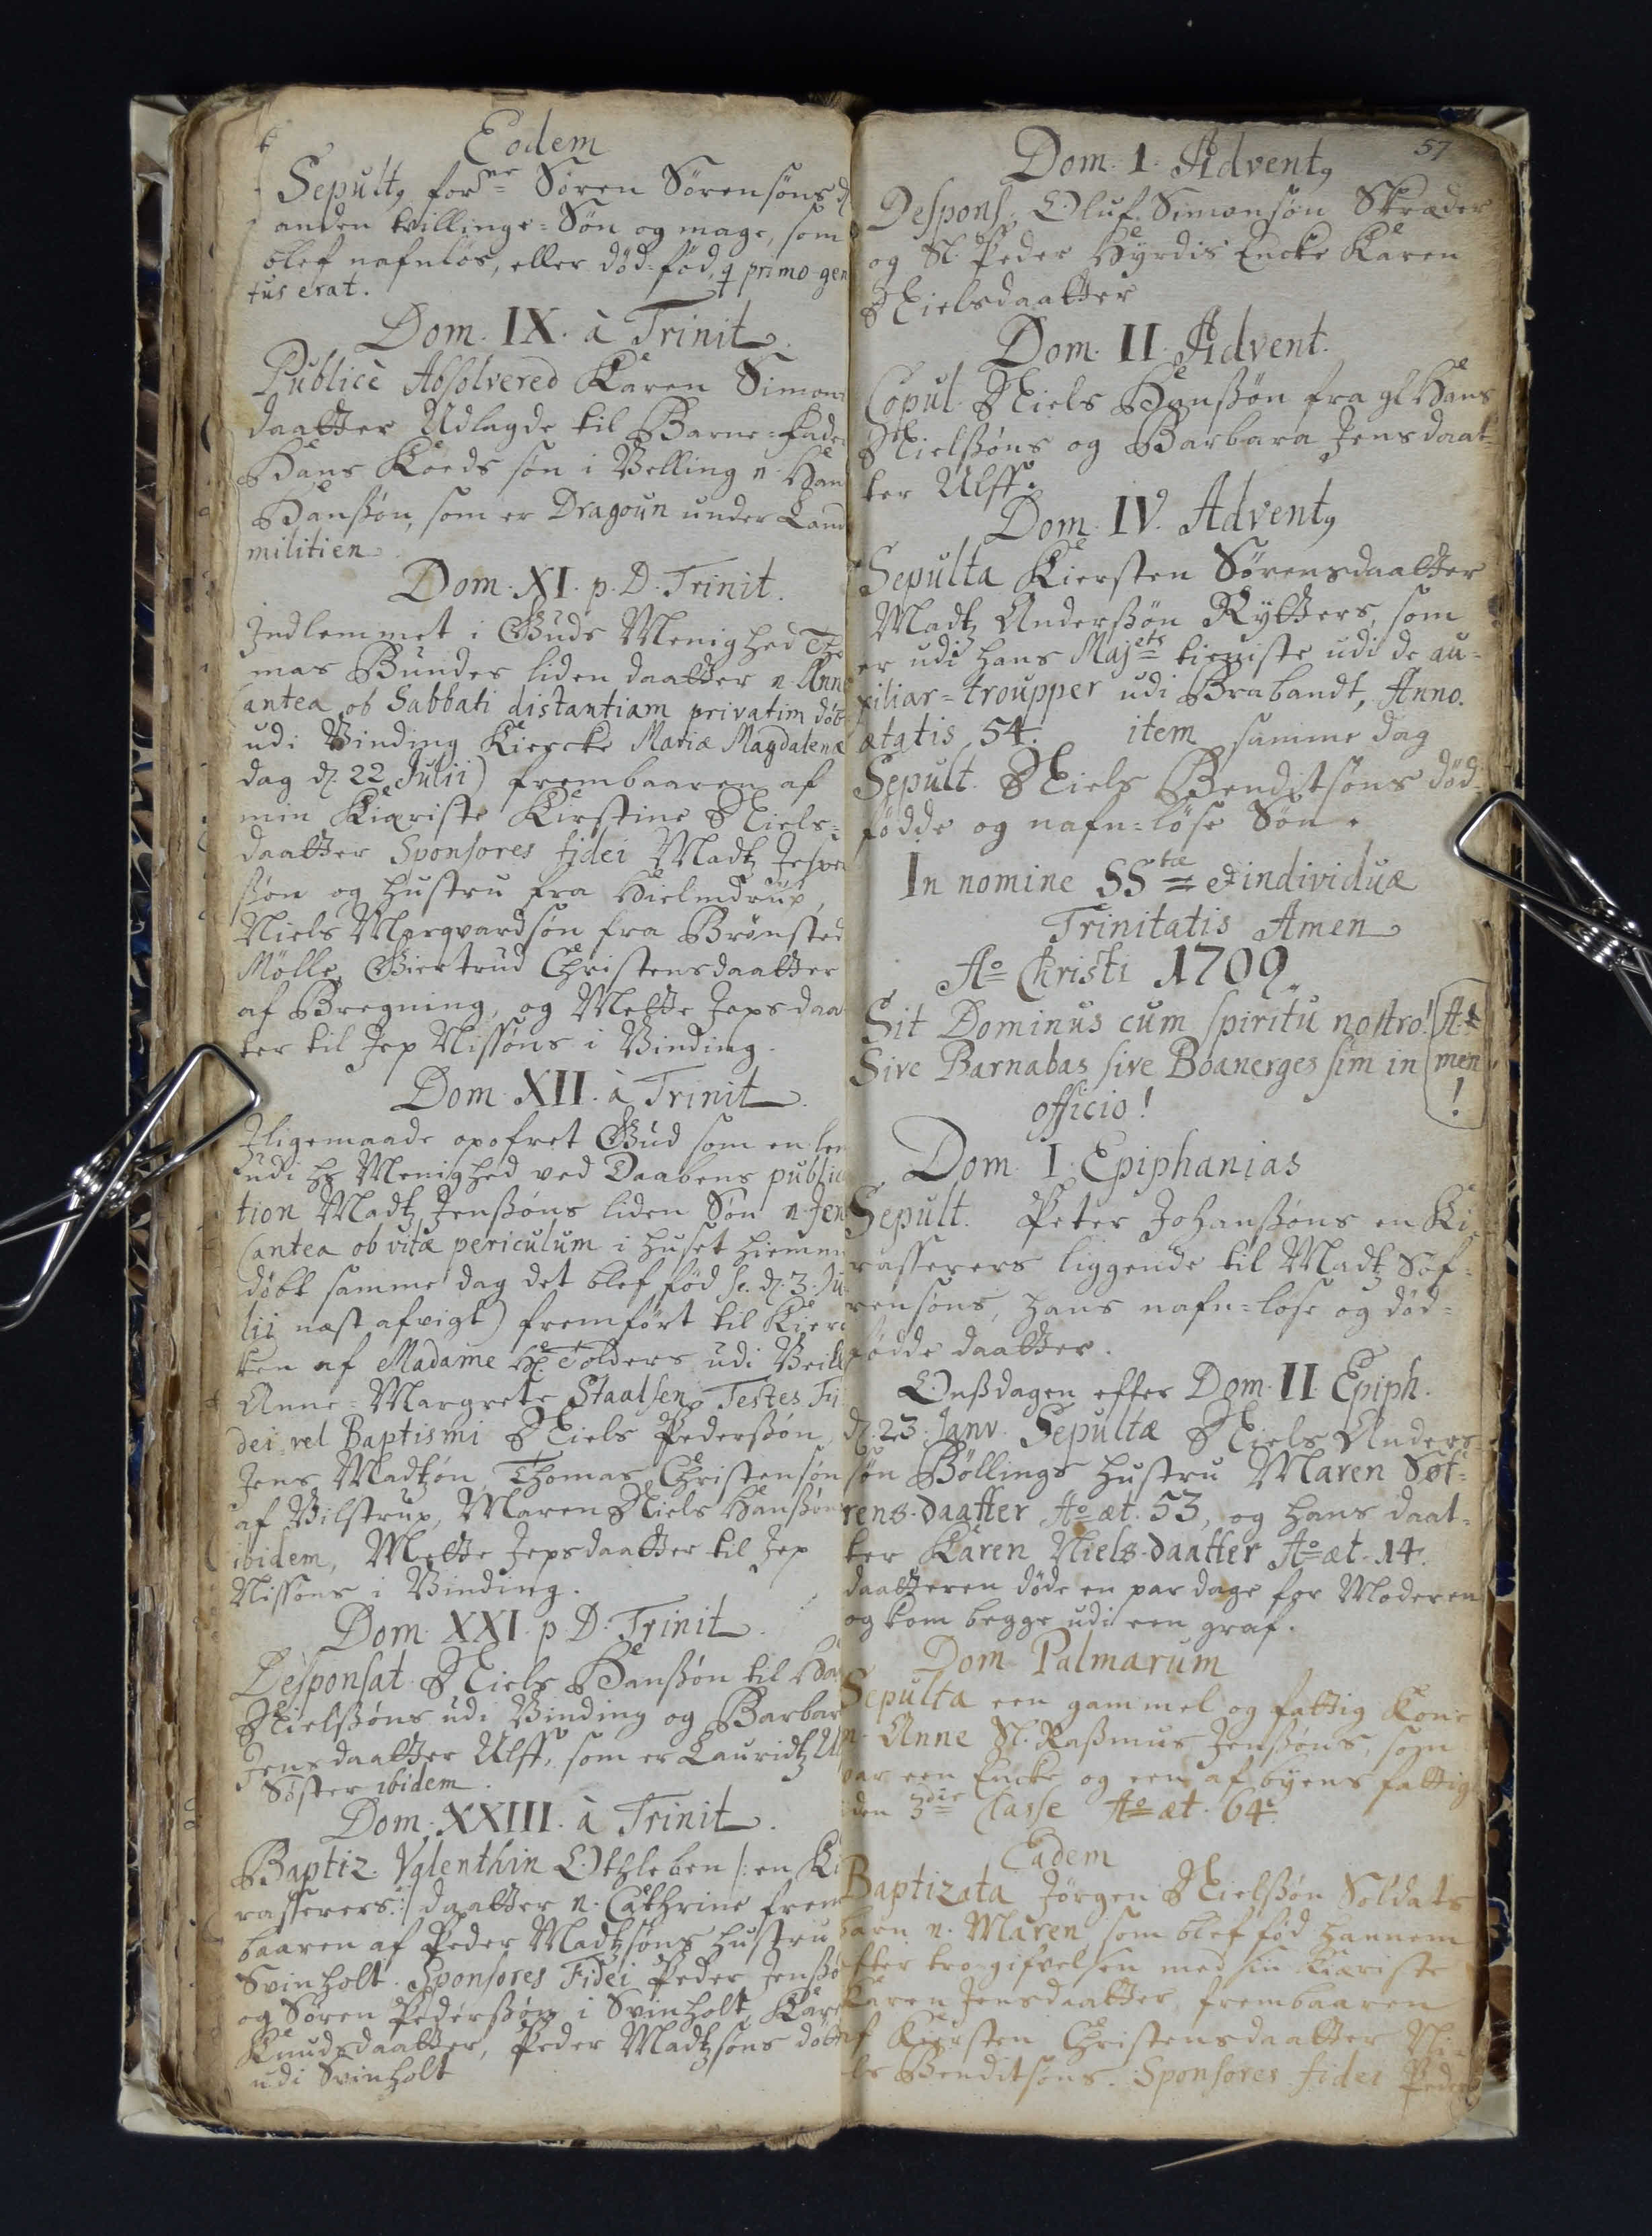Eodem
Sepultq forne Søren Sørensøns d
anden tvillinge=Søn og mage, som
blef nafnløs, eller dødfød, q primo gen
tus erat.
Dom. IX. a Trin
Publice Absolvered Karen Simon¬ 
daatter Udlagde til Barne=fade 
Hans Koeds søn i Velling n. Han 
Hanssøn, som er Dragoun under Land
militien
Dom. XI. p. D. Trinit.
Indlemmet i Guds Menighed Tho
mas Bundes liden daatter n. Ann
(antea ob Sabbati distantiam privatim døb
udi Vinding Kircke Mariæ Magdalenæ
dag d. 22 Julii) frembaaren af
min Kiæriste Kirstine Niels¬
daatter. Sponsores fidei Madtz Jesper
søn og hustru fra Hielmdrup
Niels Marqvardsøn fra Brønsted
Mølle. Giertrud Christensdaatter
af Breining, og Mette Jepsdaa 
ter til Jep Nissøns i Vinding
Dom. XII. a Trin
Iligemaade opofret Gud som en
udi hs Menighed ved Daabens public 
tion Madtz Jenssøns liden Søn n. Jen 
(antea ob vitæ periculum i huset hiemm 
døbt samme dag det blef fød sc. d. 3 Ju
lii næst afvigt) fremført til Kier
ken af Madame Hl Tolders udi Veil 
Anne =  Margrete Staalsen. Testes. Fi
dei ## Baptismi Niels Pederssøn
Jens Madtzøn, Thomas Christensøn
af Vilstrup. Maren Niels Hanssøns
ibidem, Mette Jepsdaatter til Jep
Nissøns i Vinding
Dom. XXI. p. D. Trinit
Desponsat Niels Hanssøn til Han 
Nielssøns udi Vinding og Barbar
Jensdaatter Ulff, som er Lauridtz Ul 
Søster ibidem
Dom. XXIII. a Trinit
Baptiz. Valenthin Othleben /: en Ki
rasserers.:/ daatter n. Cathrine, frem
baaren af Peder Madtzøns hustru
Svinholt. Sponsores Fidei Peder Jenssø 
og Søren Pederssøn i Svinholt. Kare 
Knudsdaatter, Peder Madtzøns døtr 
udi Svinholt
51
Dom. 1. Adventq
Despons. Oluf Simonsøn Skræder
og Sl. Peder Hyrdis Encke Karen 
Nielsdaatter
Dom. II. Advent.
Copul Niels Hanssøn fra gl Hans
Nielssøns og Barbara Jensdaat¬
ter Ulff
Dom. IV. Adventq
Sepulta Kiersten Sørensdaatter
Madtz Anderssøn Rytters, som
er udi hans Majets tieneste udi de au¬
xiliar=troupper udi Brabandt, Anno.
ætatis 54. item samme dag
Sepult. Niels Benditsøns død¬
fødde og nafn=løse Søn.
In nomine SStæ et individuæ
Trinitatis Amen
Ao Christi 1709
Sit Dominus cum spiritu nostro A¬
men
Sive Barnabas sive Boanerges sim in
ofsicio!
Dom. I. Epiphanias
Sepult. Peter Johanssøns en Ki¬
rasserers liggende til Madtz Søf¬
rensøns, hans nafn=løse og død¬
fødde daatter
Onsdagen efter Dom. II. Epiph.
d. 23 Janv Sepultæ Niels Anders
søn Bøllings hustru Maren Søf¬
rensdaatter Ao æt. 53, og hans daat¬
ter Karen Niels-daatter Ao æt. 14.
daatteren døde en par dage for Moderen
og kom begge udi een graf.
Dom. Palmarum.
Sepulta een gammel og fattig Kone
n. Anne Sl. Rassmus Jenssøns, som
var een Encke og een af byens fattige
den 3die Classe Ao æt. 64
Eadem
Baptizata Jørgen Nielssøn Soldats
barn n. Maren, som blef fød hannem
fter tro gifvelsen med sin Kiæriste
Karen Jensdaatter frembaaren
af Kiersten Christensdaatter Nie
ls Benditsøns. Sponsores fidei Peder